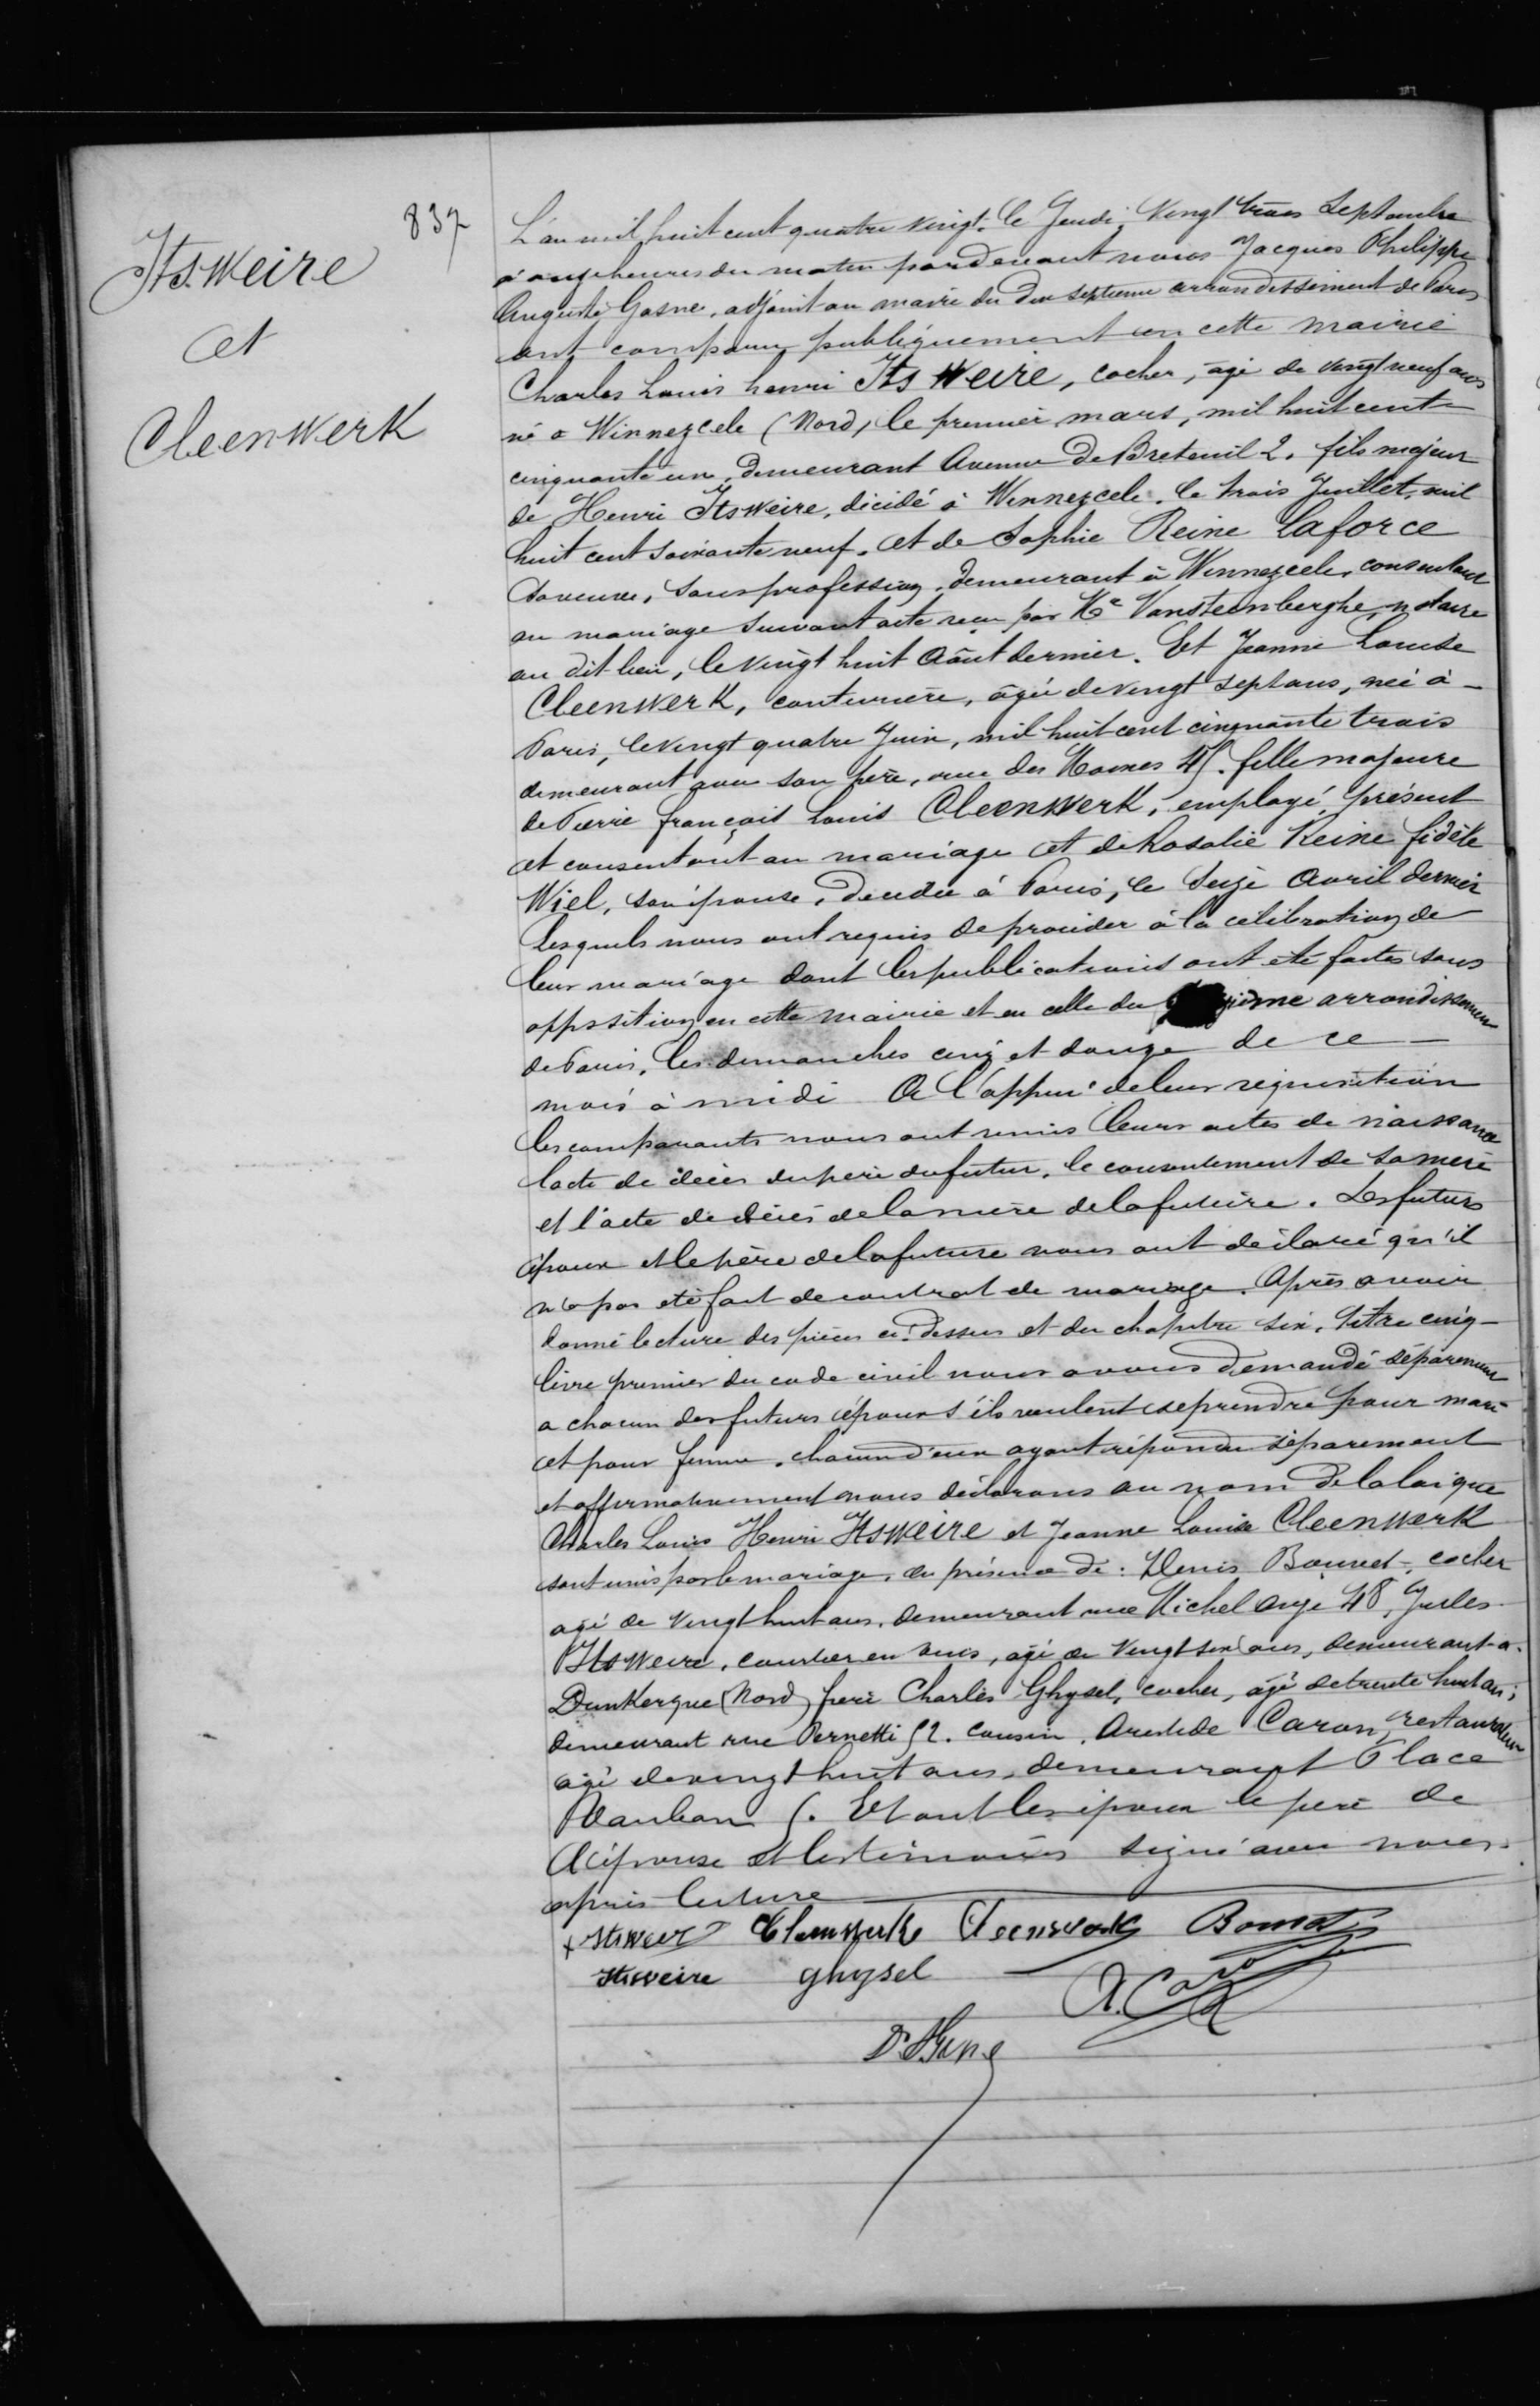837
Itsweire
et
Cleenwerk
L'an mil huit cent quatre vingt le Jeudi vingt trois Septembre
cinq heures du matin par devant nous Jacques Philippe
Auguste Gasne, adjoint au maire du dix septième arrondissement de Paris
ont comparu publiquement en cette mairie
Charles Louis henri Itsweire, cocher, âge de vingt neuf ans
né à Winnezeele (Nord), le premier mars, mil huit cent
cinquante un, demeurant Avenue de Breteuil 2, fils majeur
de Henri Itsweire, décédé à Wennezeele. le trois Juillet, mil
huit cent soixante neuf, et de Sophie Reine Laforce
sa veuve, sans profession. demeurant à Wennezeele, consentant
au mariage suivant acte reçu par M° Vantenberghe, notaire
au dit-lieu, le vingt huit Août dernier. Et Jeanne Louise
Cleenwerk, couturière, âgée de vingt sept ans, née à -
Paris, le vingt quatre Juin, mil huit cent cinquantre trois
demeurant avec son père, rue des Moines 48. fille majeure
de Pierre françois Louis Cleenwerk, employé, présent
et consentant au mariage et de Rosalie Reine fidèle
Wiel, son épouse, décédée à Paris, le Seize Avril dernier
Lesquels nous ont requis de procéder à la célébration de
leur mariage dont les publications ont été faite sans
opposition en cette mairie et en celle du Quinzième arrondissement
de Paris. les dimanches cinq et douze de ce -
mois à midi A l'appui de leur requisition
les comparant nous ont remis leurs actes de naissance
l'acte de décès du père du futur, le consentement de sa mère
et l'acte de décès de la mère de la future. Les futurs
époux et le père de la future nous ont déclaré qu'il
n'a pas été fait de contrat de mariage. Après avoir
donné lecture des pièces ci-dessus et du chapitre six, Titre cinq-
livre premier du code civil nous avons demandé séparément
a chacun des futurs époux s'ils veulent se prendre pour mari
et pour femme. chacun d'eux ayant répondu séparément
et affirmativement nous déclarons au nom de la loi que
Charles Louis Henri Itsweire et Jeanne Louis Cleenwerk
sont unis par le mariage en présence de Henri Bonnet-cocher
agé de vingt huit ans, demeurant rue Michel agé 48, Jules
Itsweire, courtier en cuir, agé de Vingt six ans, demeurant a
Dunkerque (Nord) frere Charles Ghysel, cocher, âgé de trente huit ans;
demeurant rue Pernetti 52, cousin, Duerte de Caron, restaurateur
agé de vingt huit ans demeurant Place
Vauban 5. Et ont les époux le père de
l'épouse et les témoins signé avec nous
après lecture-
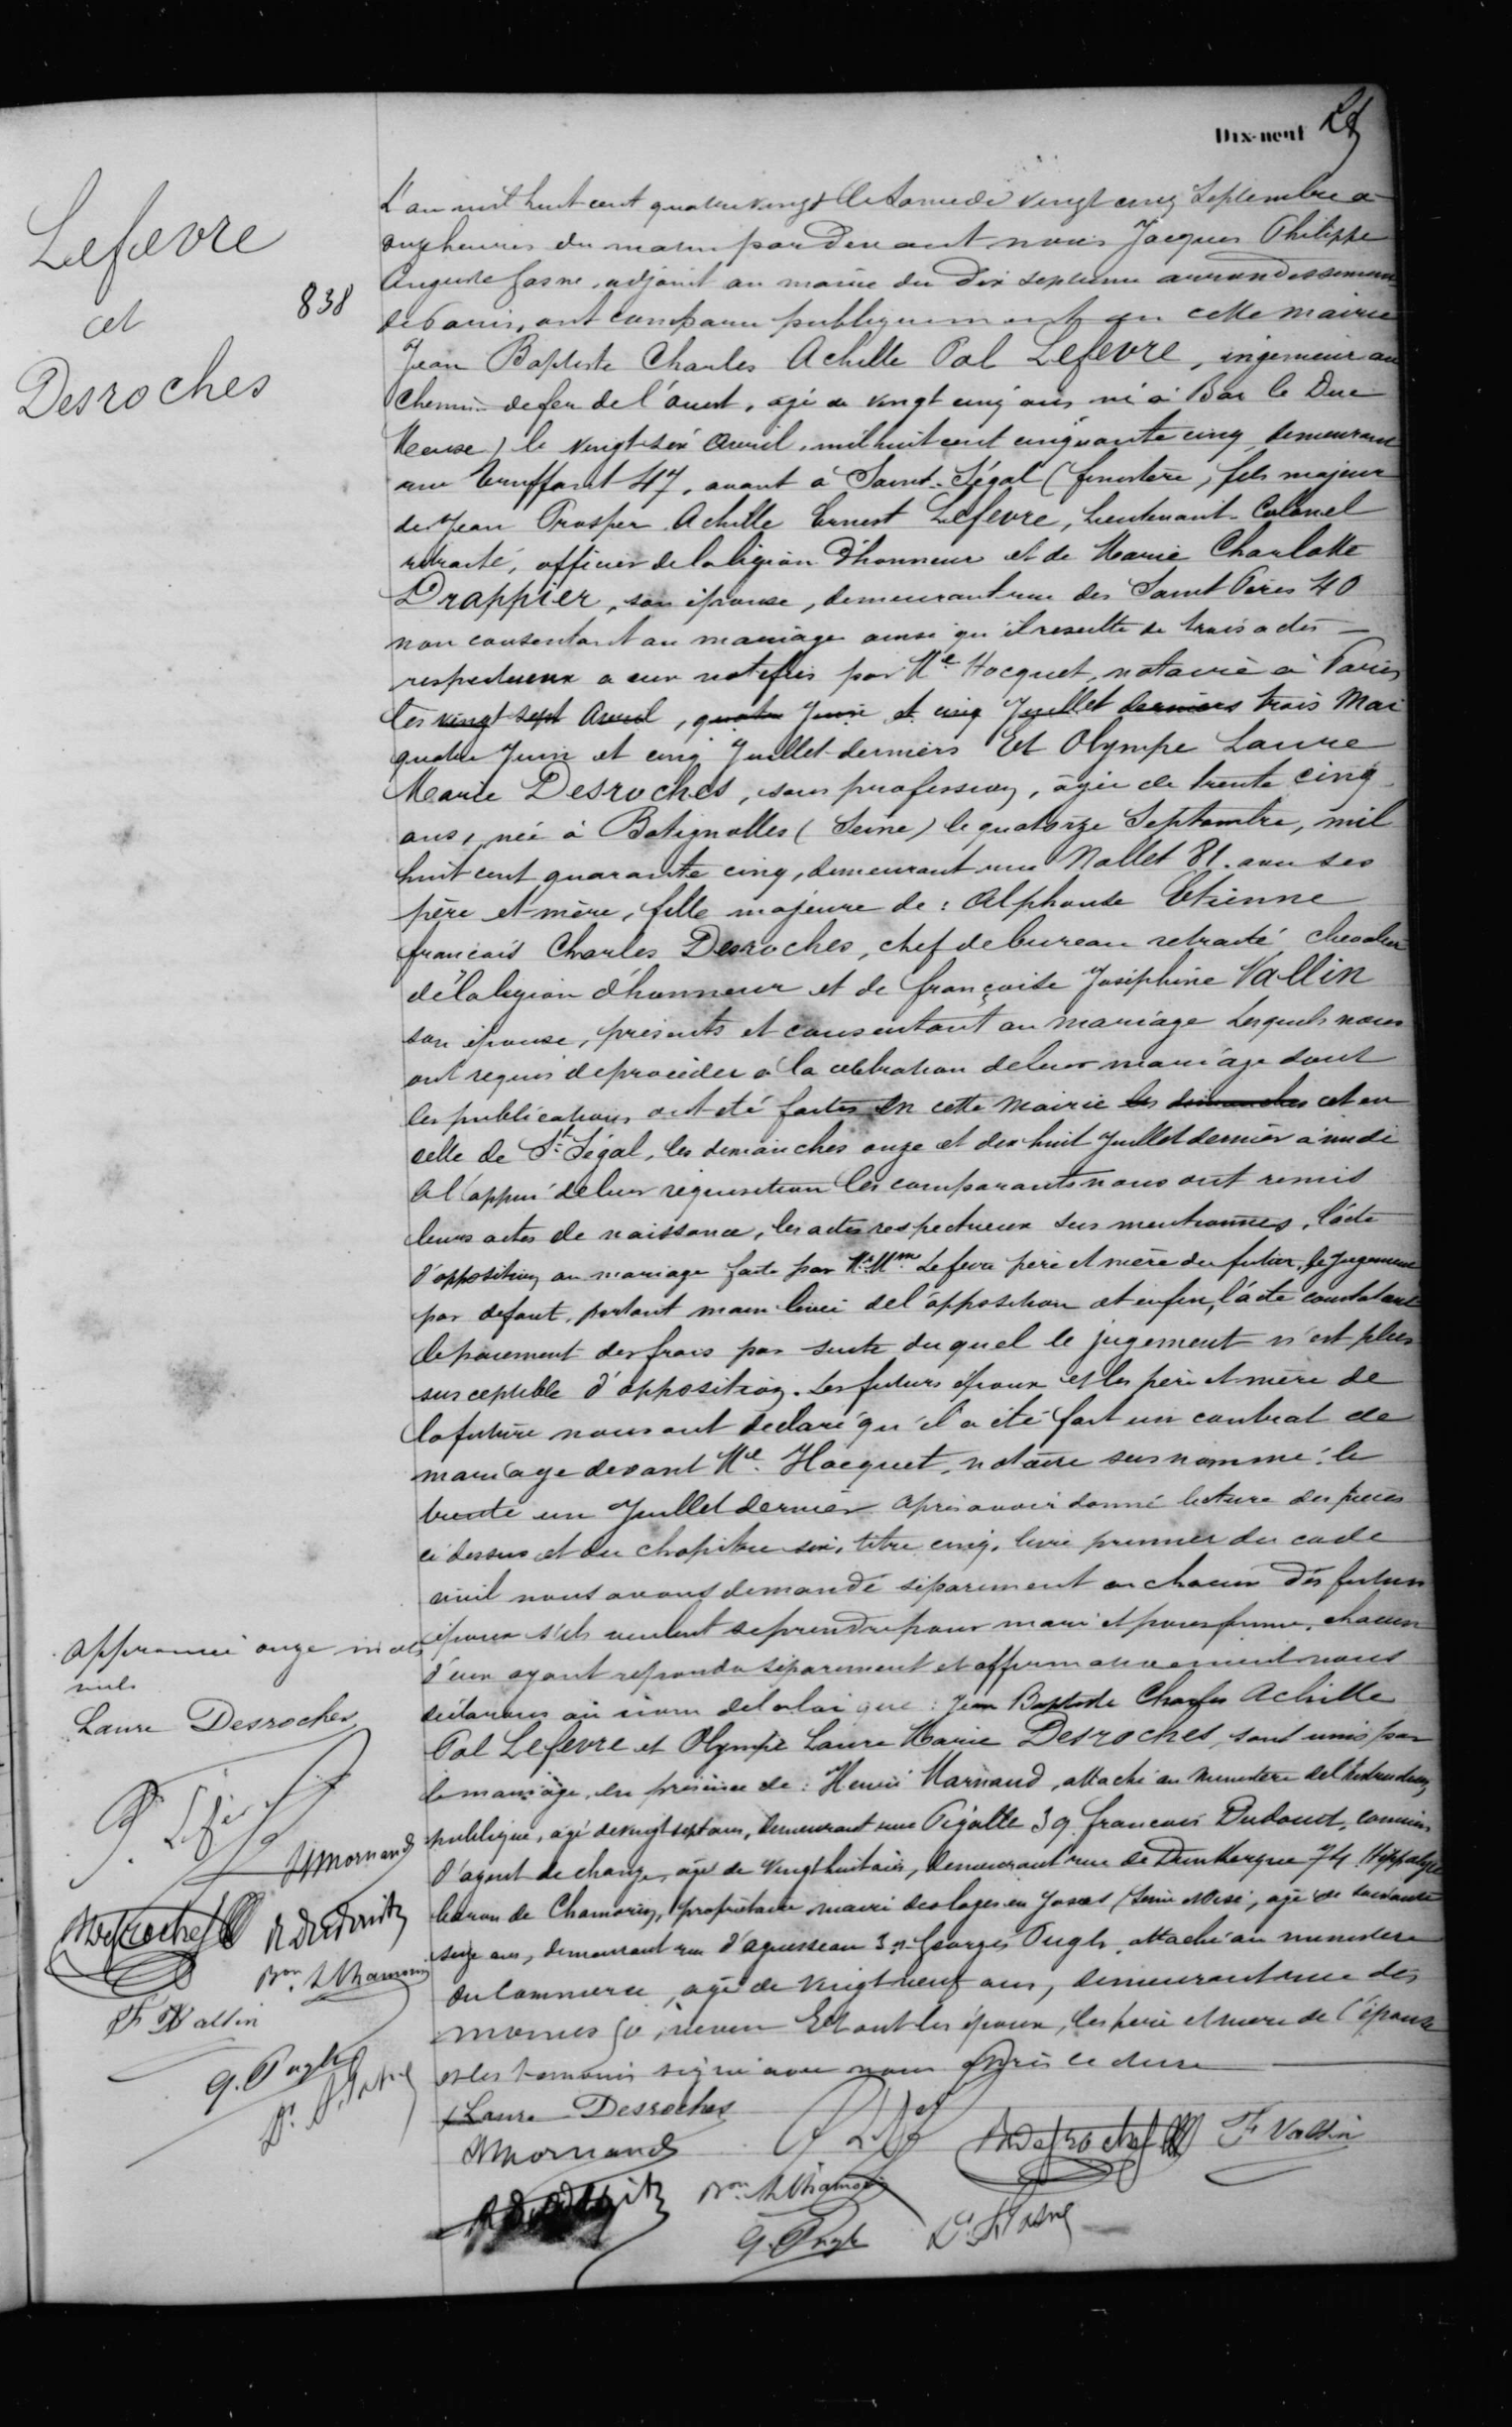Lefevre
et 838
Desroches
L'an mil huit cent quatre vingt le samedi vingt cinq Septembre a-
onze heures du matin par devant nous Jacques Philippe
Auguste Gasne, adjoint au maire du dix septième arrondissement
de Paris, ont comparu publiquement en cette mairie
Jean Baptiste Charles Achille Pol Lefevre, ingénieur au
Chemin de fer de l'ouest, agé de vingt cinq ans né à Bar le Duc
Meuse) le vingt six avril mil huit cent cinquante cinq, demeurant
rue Truffaut 47, avant à Saint Ségal (finistère, fils majeur
de Jean Prosper Achille Ernest Lefevre, Lieutenant Colonel
retraité, officier de la légion d'honneur et de Marie Charlotte
Drappier, son épouse, demeurant rue des Saint Pères 40
non consentant au mariage ainsi qu'il résulte de trois actes
respectueux à eux notifiés par Me Hocquet, notaire à Paris
Les vingt sept Avril, quatre Juin et cinq Juillet derniers trois Mai
quatre Juin et cinq Juillet derniers Et Olympe Laure
Marie Desruches, sans profession, âgés de trente cinq
ans, née à Batignolles (Seine) le quatorze Septembre, mil
huit cent quarante cinq, demeurant rue Mallet 81, avec ses
père et mère, fille majeure de: Alphonse Etienne
français Charles Desroches, chef de bureau retraité chevalier
de la légion d'honneur et de françoise Joséphine Vallin
son épouse, présents et consentant au mariage Lesquels nous
ont requis de procéder à la célébration de leur mariage dont
les publications ont été faites en cette mairie les dimanches cet en
celle de St Ségal, les dimanches onze et dix huit Juillet dernier à midi
de l'appui de leur réquisition les comparants nous ont remis
leurs actes de naissance, les actes respectueux sus mentionnées, l'acte
d'opposition, au mariage fait par Mm Lefevre père et mère du futur, le jugement
par defaut, portant main levée de l'opposition et enfin, l'acte constatant
le paiement des frais par suite duquel le jugement n'est plus
susceptible d'opposition. Les futurs époux et les père et mère de
la future nous ont déclaré qu'il a été fait un contrat de
mariage devant Me Hacquet, notaire sus nommé: le
trente un Juillet dernier, après avoir donné lecture des pièces
ci dessus et du chapitre six, titre cinq, livre premier du code
civil nous avons demandé séparement a chacun des futurs
époux s'ils veulent se prendre pour mari et pour femme, chacun
d'eux ayant répondu séparement et affirmativement nous
déclarons au nom de la loi que: Jean Baptiste Charles Achille
Pol Lefevre et Olymbe Laure Marie Desroches sont unis par
le mariage, en présence de Henri Mariaud, attaché au ministère de l'instruction
publique, agé de vingt sept ans, demeurant rue Pigalle 39. francois Dudoud, coursier
d'agent de change, agé de vingt huit ans, demeurant rue de Dunkerque 74, Hippolyte
lecron de Chamarin, propriétaire, maire des loges en Josas (Seine et Oise, agé de soixante
seize ans, demeurant rue d'Aguesseau 3, George Ough, attaché au ministère
du commerce, agé de vingt neuf ans, demeurant rue des
mornes 50, neveu. Et ont les époux, les père et mère de l'épouse
et les témoins signé avec nous après le lecture-